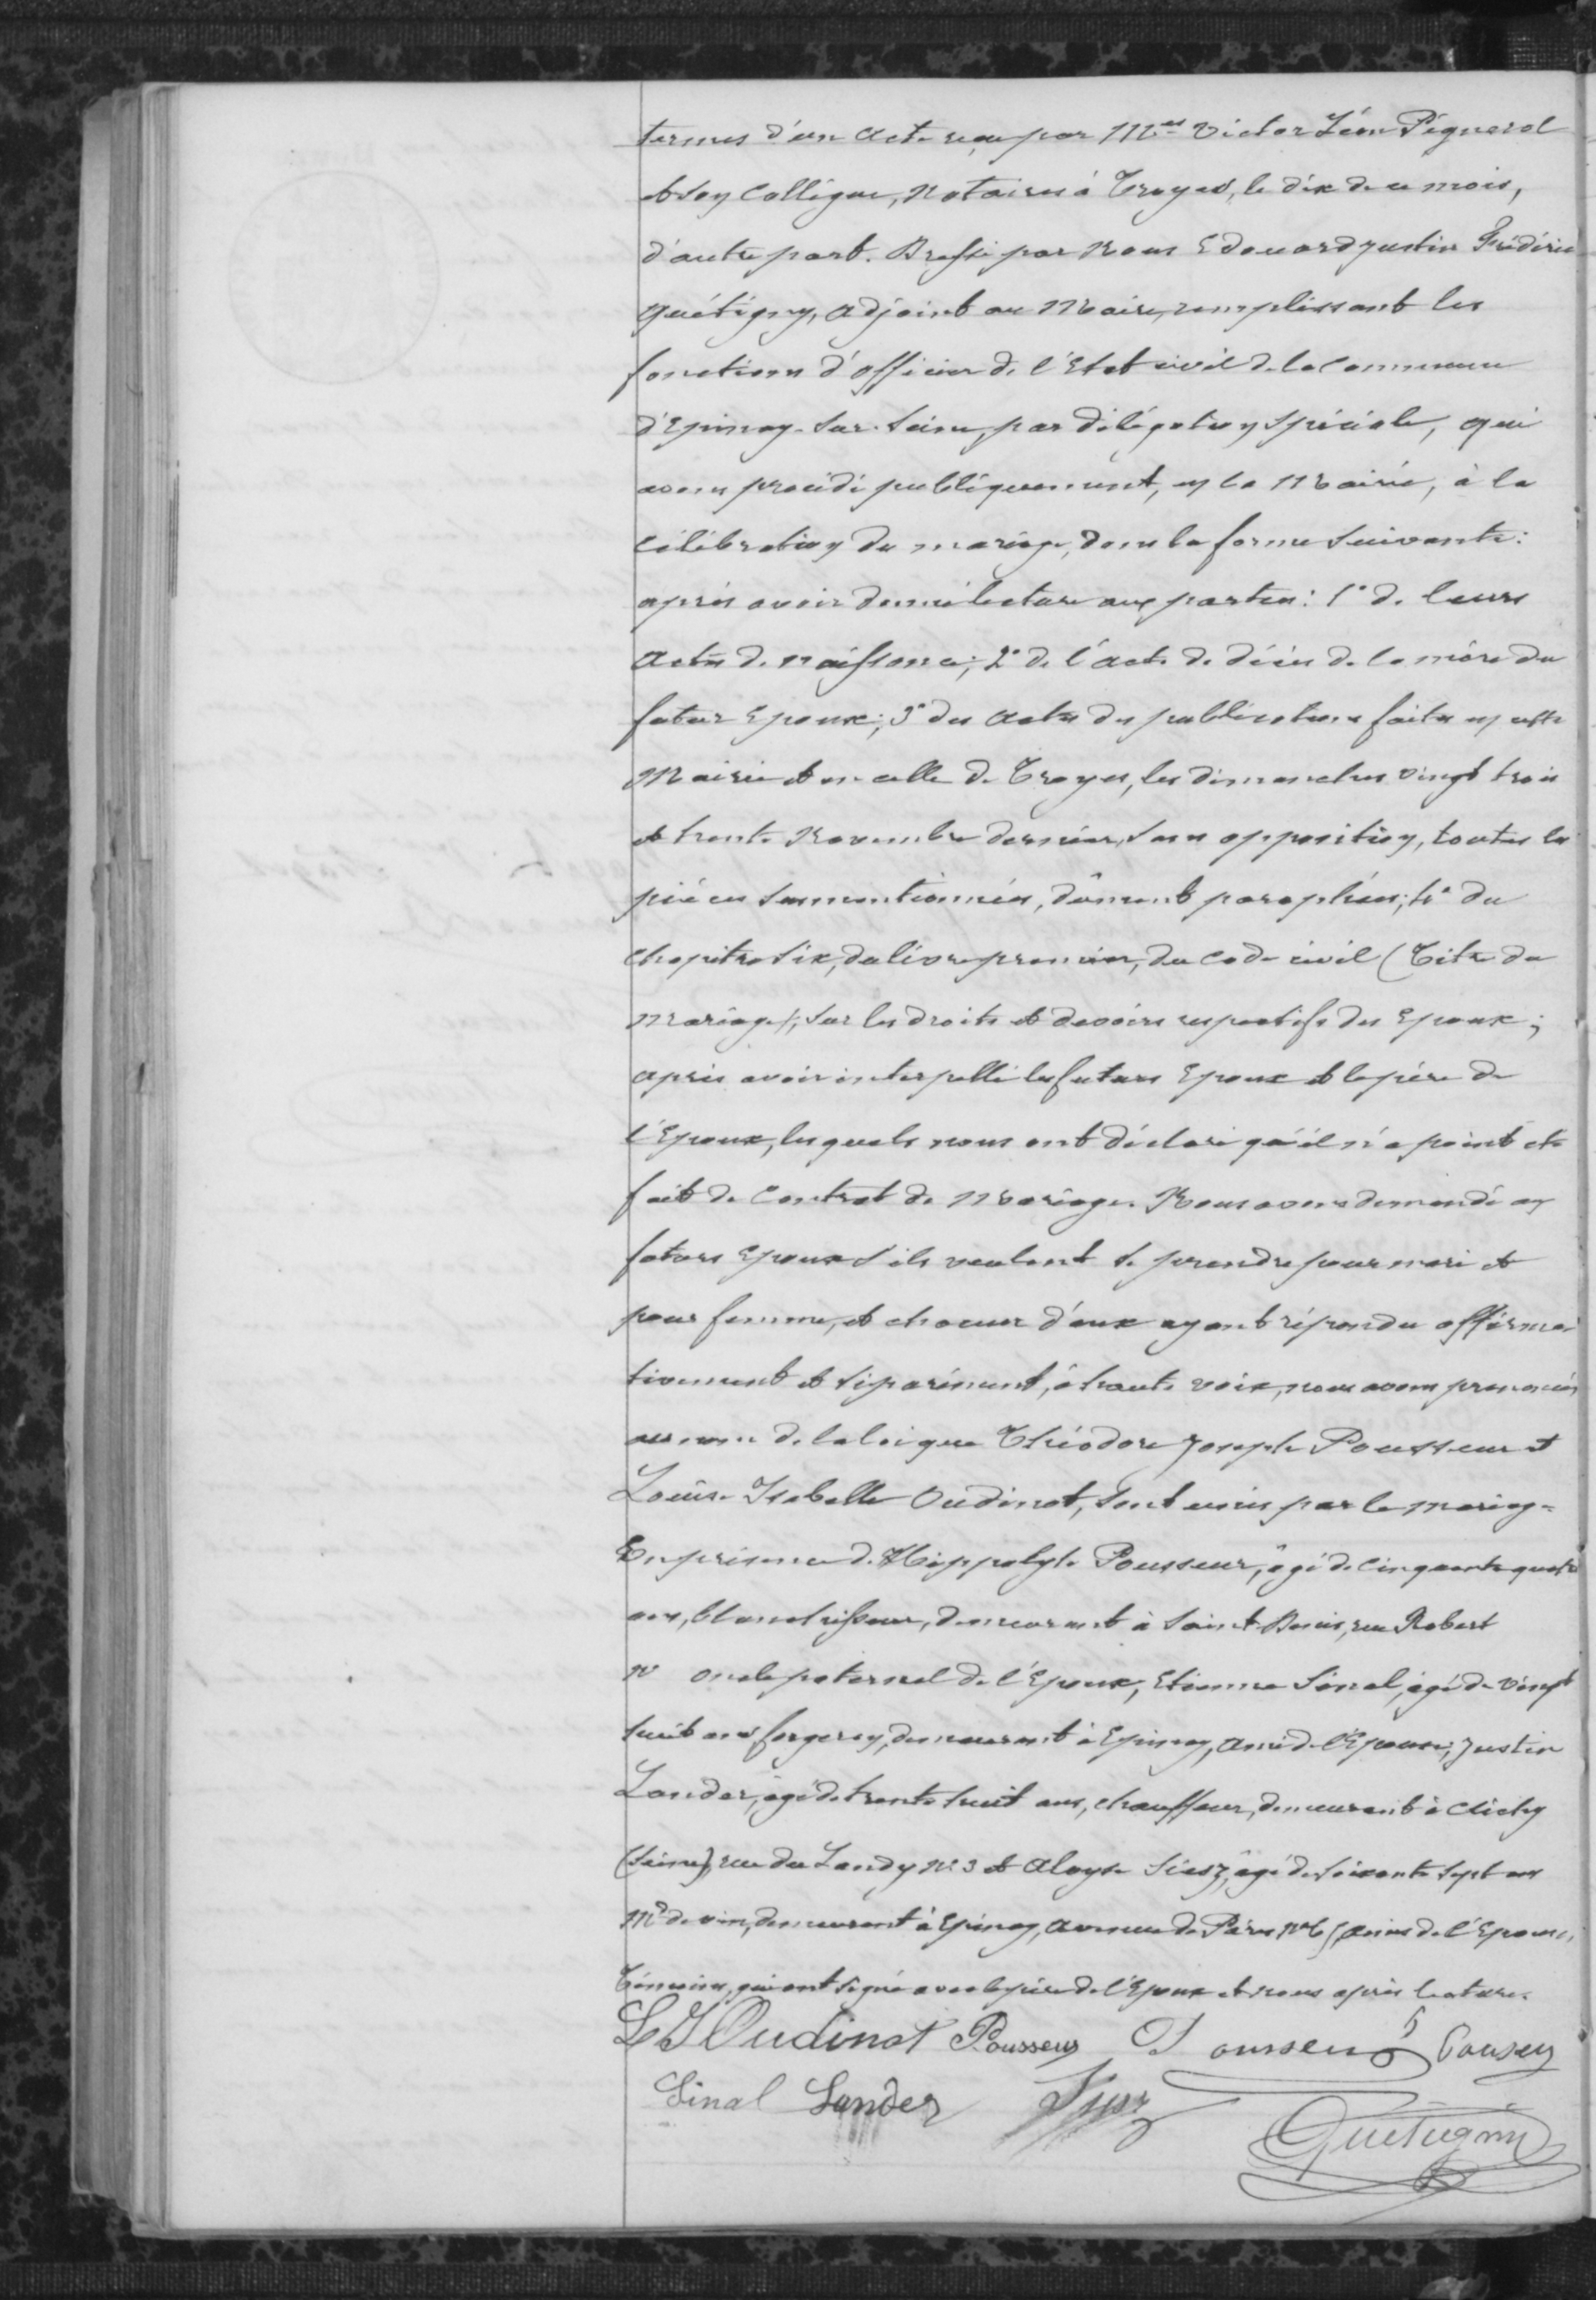termes d'un acte reçu par Me Victor Léon Pignorol
nb son collègue, notaire à Troyes, le dix de ce mois,
d'autre part. Dressé par nous Edouard Justin Frédéric
Quétigny, adjoint au maire, remplissant les
fonctions d'officier de l'Etat civil de la commune
d'Epinay-sur-Seine par délégation spéciale, qui
avons procédé publiquement, en la Mairie, à la
célébration du mariage, dans la forme suivante:
après avoir donné lecture aux parties: 1° de leurs
actes de naissance; 2° de l'acte de décès de la mère du
futur époux 3° des actes de publication faites en cette
Mairie et en celle de Troyes, les dimanches vingt trois
et trente novembre dernier sans opposition, toutes les
pièces susmentionnées, dûment paraphées; 4° du
chapitre six, du livre premier, du code civil (Titre du
mariage); sur les droits et devoirs respectifs des epoux;
après avoir interpellé les futurs epoux et le père de
l'epoux, lesquels nous ont déclaré qu'il n'a point été
fait de contrat de mariage. Nous avons demandé aux
futurs epoux s'ils veulent se prendre pour mari et
pour femme, et chacun d'eux ayant répondu affirma
tivement et séparément, à haute voix, nous avons prononcé
au nom de la loi que Théodore Josephe Pousseau et
Louis Isabelle Oudinot, sont unis par le mariage
En présence d'Hippolyte Pousseur, âgé de cinquante quatre
ans, blanchisseur, demeurant à Saint Denis, rue Robert
oncle paternel de l'epoux, Etienne Simal, âgé de vingt
huit ans forgeron, demeurant à Epinay, ami de l'Epoux; Justin
Londer, âgé de trente huit ans, chauffeur, demeurant à Clichy
(Seine) rue du Landy 249 et Aloye Siesz, âgé de soixante sept ans
Md de vin, demeurant à Epinay, avenue de Paris N°6 (Seine de l'epouse
témoins qui ont signé avec le père de l'epouse et nous après lecture
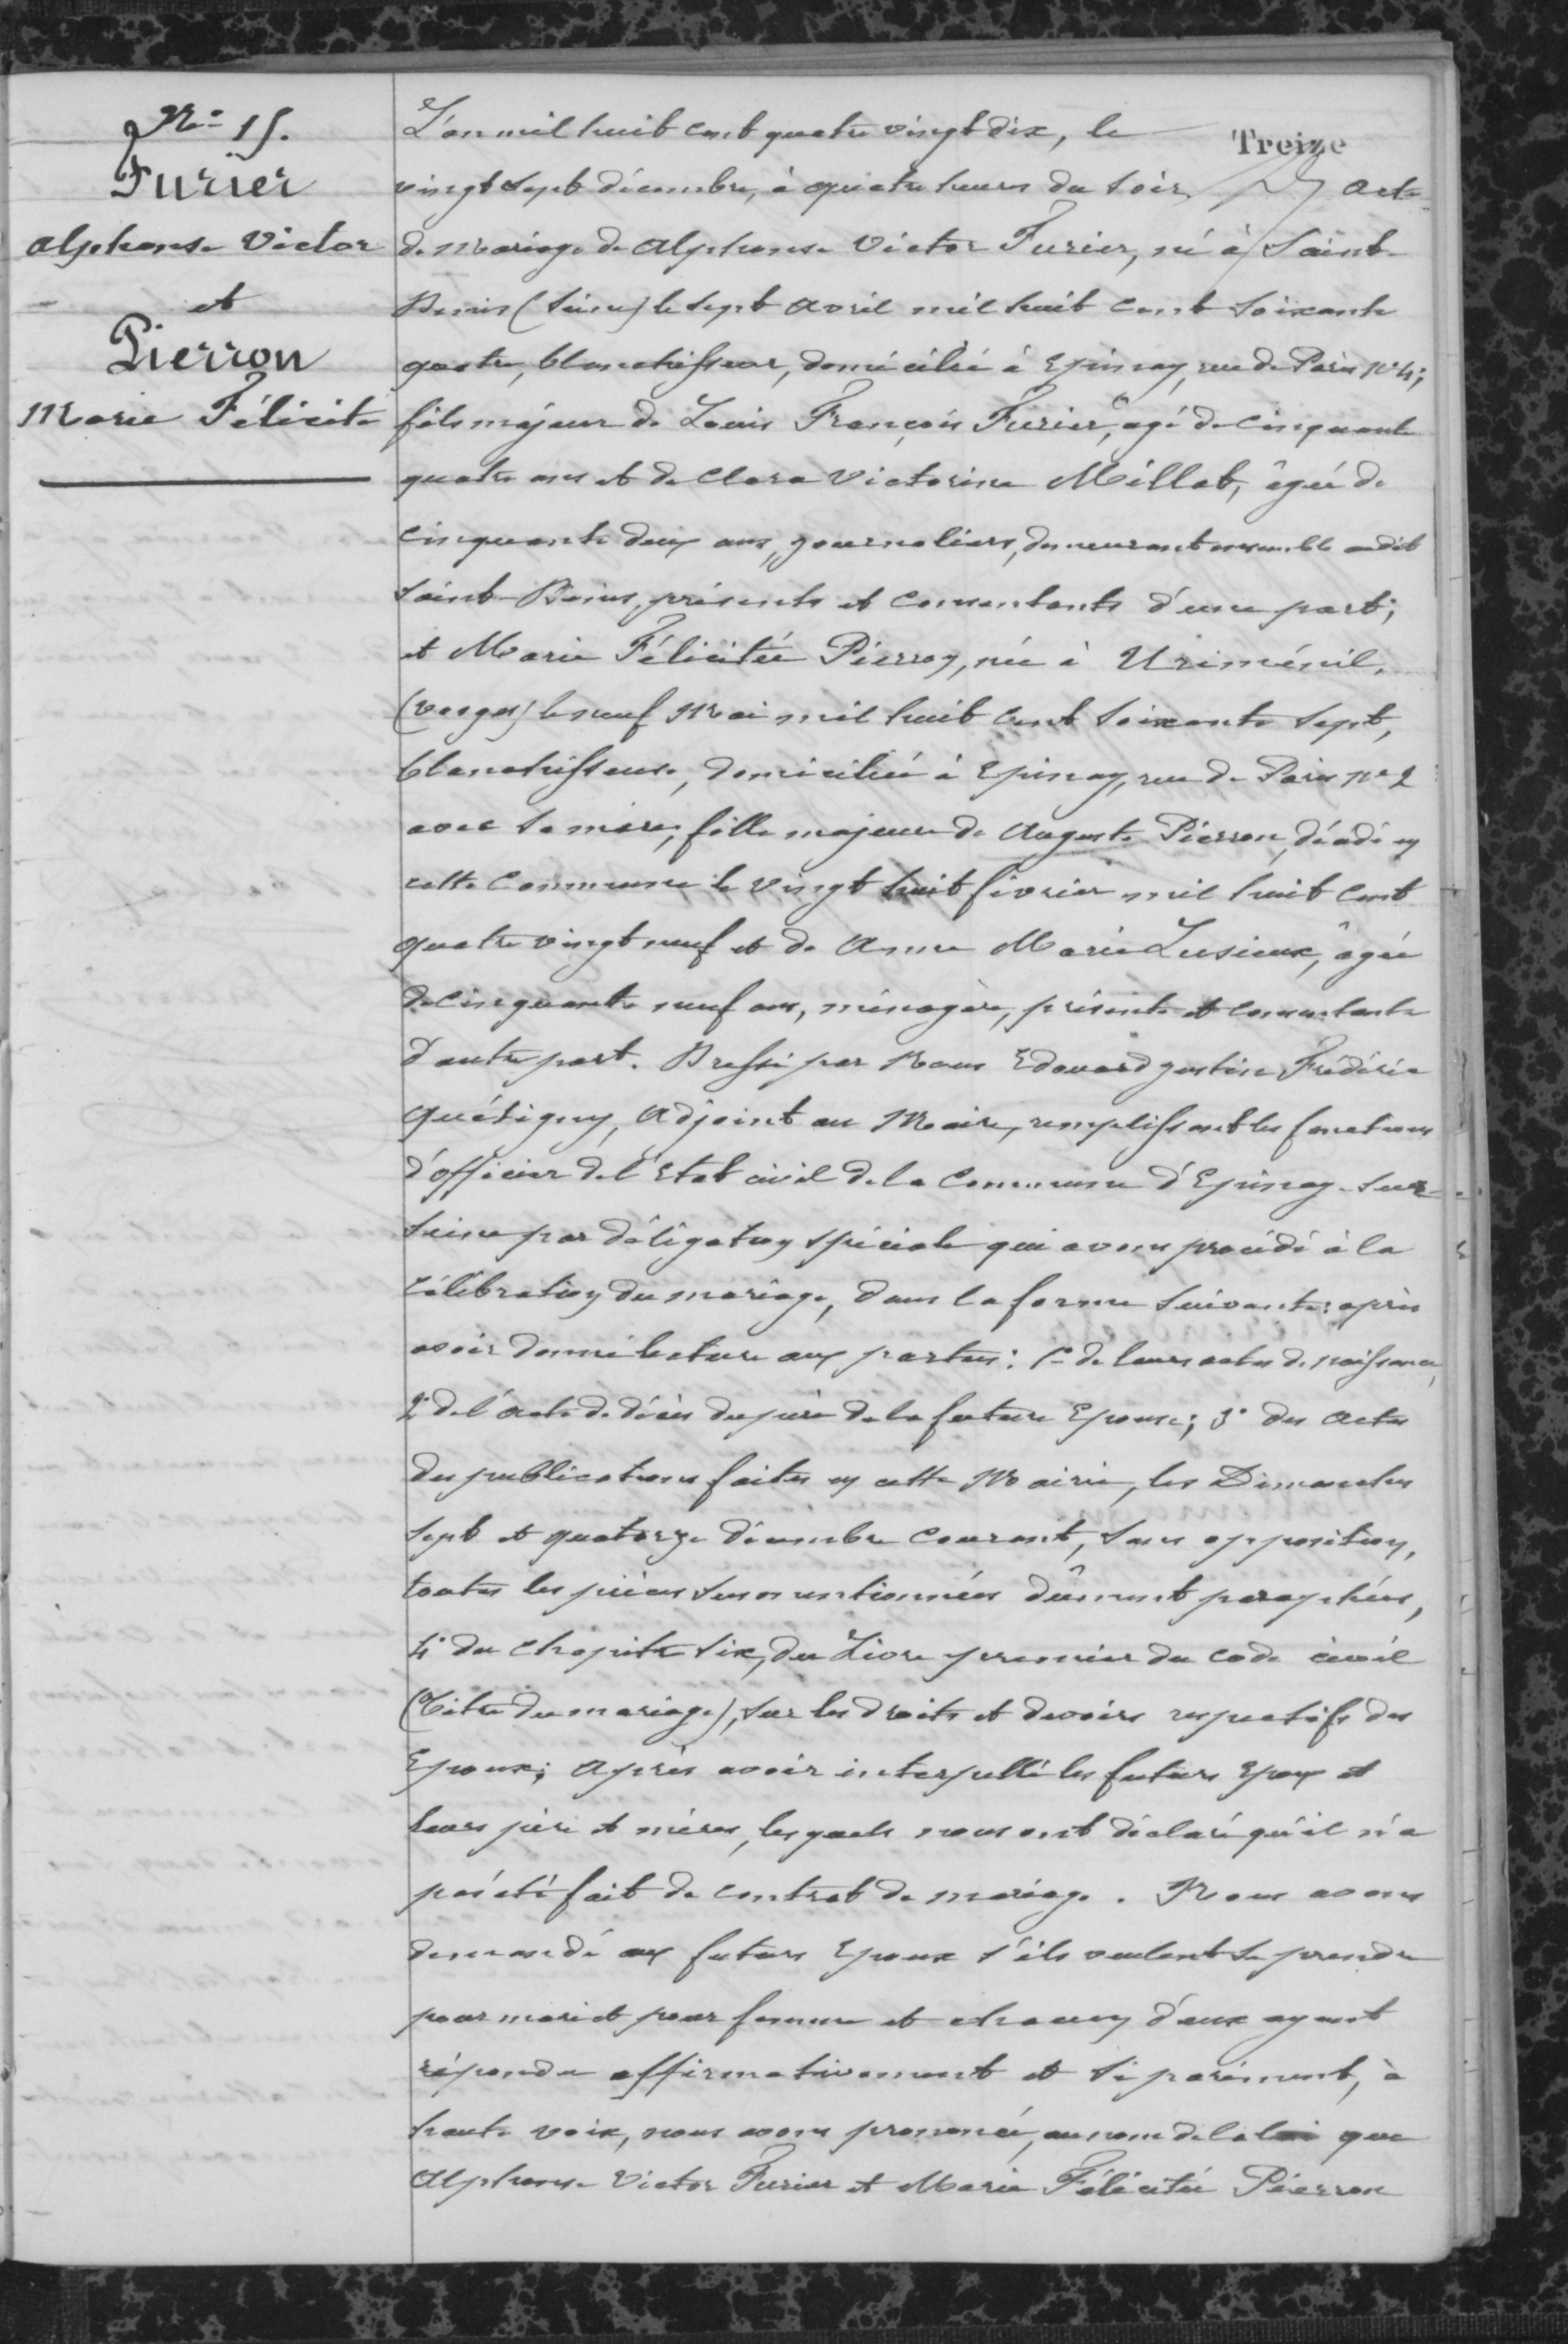N°15
Furier
Alphonse Victor
et
Pierron
Marie Félécité
L'an mil huit cent quatre vingt dix, le
vingt sept décembre, à quinze heures du soir, acte
de mariage de alphonse Victor Furier, né à Saint
Denis -Seine) le sept avril mil huit cent soixante
quatre, blanchisseur, domicilié à Epinay, rue de Paris N°4;
fils majeur de Louis François Furier, âgé de cinquante
quatre ans et de Clara Victorine Millet, âgé de
cinquante deux ans, journaliere, demeurant ensemble audit
Saint Denis, présents et consentants d'une part;
et Marie Félicitée Pierron, née à Uriménil,
(Vosges) le neuf Mai mil huit cent soixante sept,
blanchisseur, domiciliée à Epinay, rue de Paix N°2
avec la mère; fille majeur de Auguste Pierron, décédé en
cette commune le vingt huit février mil huit cent
quatre vingt neuf et de Anne Marie Lusieux, âgée
de cinquante neuf ans, ménagère, présents et consentants
d'une part. Dressé par nous Edouard gautier Frdéric
Guétigny, adjoint au Maire, remplissant les fonctions
d'officier de l'Etat civil de la Commune d'Epinay-sur-
Seine par délégation spéciale qui avons procédé à la
célébration du mariage, dans la forme suivante: après
avoir donné lecture aux parties: 1°-de leurs actes de naissance
2° de l'acte de décès du père de la future epouse; 3° des actes
des publications faites en cette Mairie, les Dimanches
sept et quatorze décembre courant, sans opposition,
toutes les pièces sus mentionnées dûment paraphées,
4° du chapitre six, du Livre premier du code civil
(Titre du mariage), sur les droits et devoirs respectifs des
Epoux; après avoir interpellé les futurs epoux et
leurs père et mère, lesquels nous ont déclaré qu'il n'a
pas été fait de contrat de mariage. Nous avons
demandé aux futurs epoux s'ils veulent se prendre
pour mari et pour femme et chacun d'eux ayant
répondu affirmativement et séparément, à
haute voix, nous avons prononcé au nom de la loi que
Alphonse Victore Furier et Maria Félécité Pierron